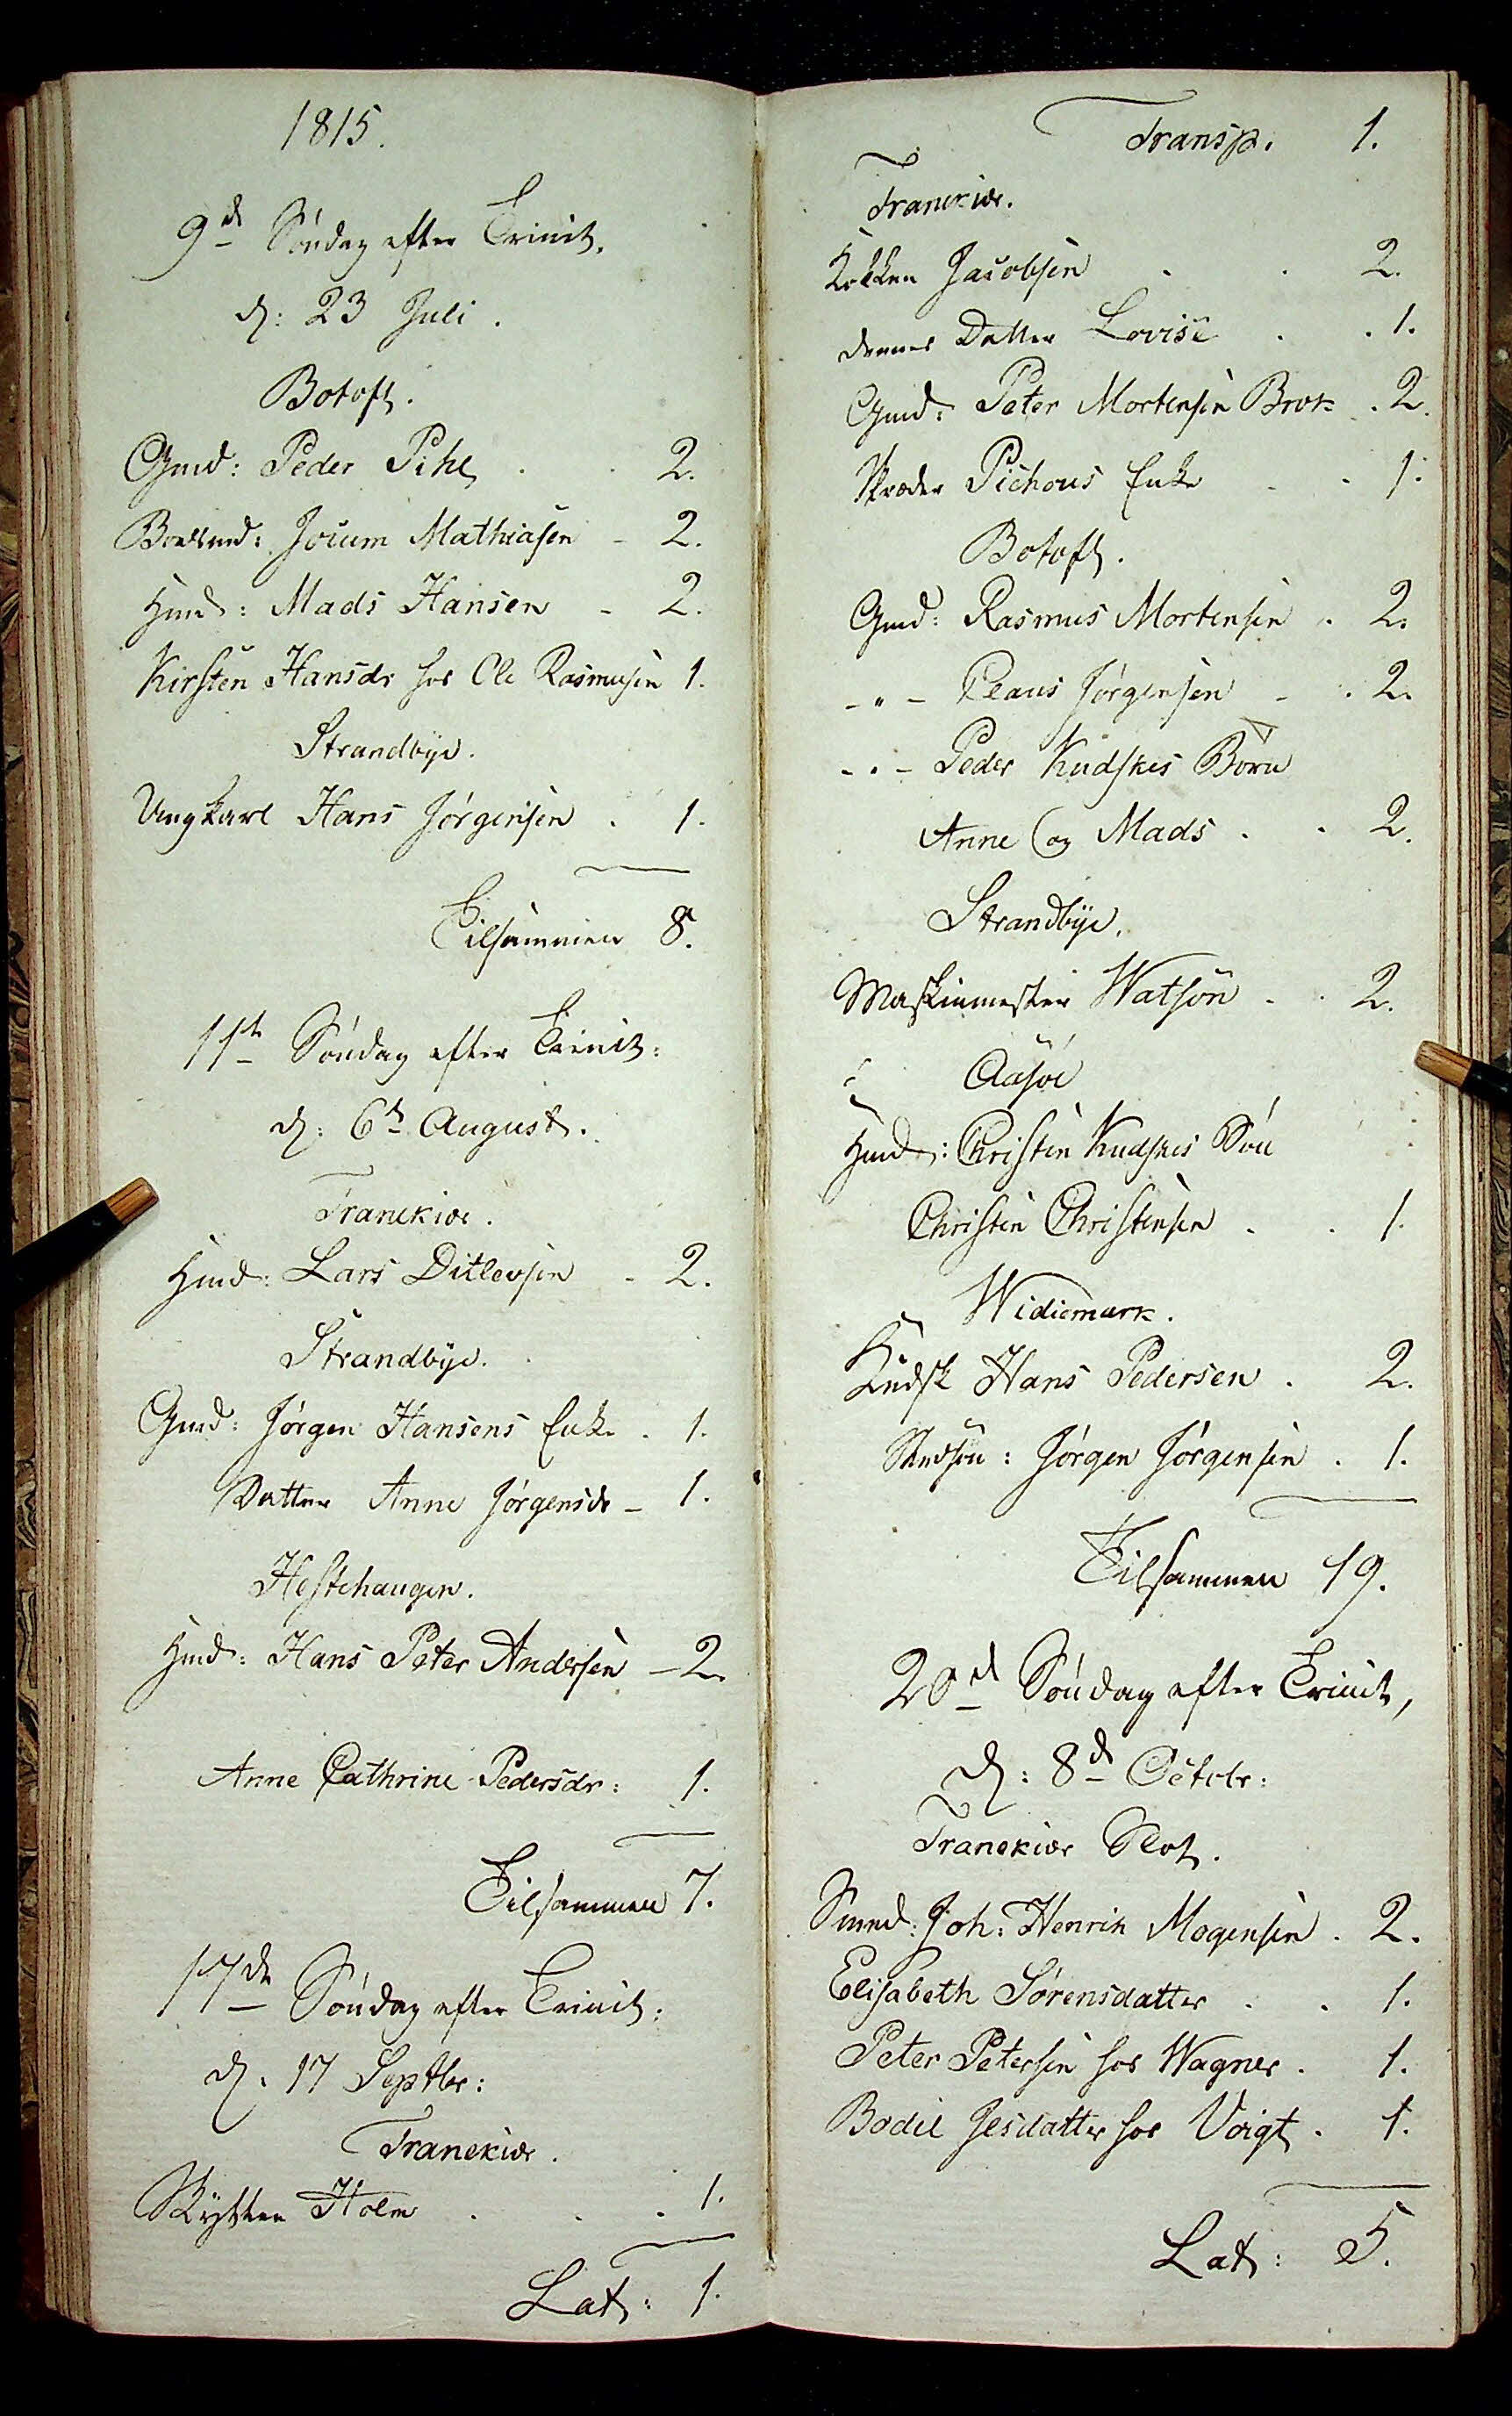1815.
9de Søndag efter Trinit.
d: 23 Juli.
Botoft
Gmd: Peder Pihl . . 2.
Boelsmd: Jocum Mathiasen - 2.
Hmd: Mads Hansen - 2.
Kirsten Hansdr hos Ole Rasmusen 1.
Strandbye.
Ungkarl Hans Jørgensen .1
Tilsammen 8.
11te Søndag efter Trinit:
d: 6te August.
Tranekiær
Hmd: Lars Ditlevsen - 2.
Strandbye
Gmd: Jørgen Hansens Enke . 1.
Datter Anne Jørgensdr -1.
Hestehaugen.
Hmd: Hans Pater Andersen - 2
Anne Cathrine Pedersdr: 1
Tilsammen 7.
17de Søndag efter Trinit:
d: 17 Septbr:
Tranekiær.
Skytten Holm . . . 1.
Lat: 1
Transp: 1.
Tranekiær.
Kokken Jacobsen . . 2.
deres Datter Lovises . . 1.
Gmd: Peder Mortensen Brok . 2
Skræder Pichous Enke . . 1.
Botoft
Gmd: Rasmus Mortensen . 2.
-"- Claus Jørgensen - . 2.
-"- Peder Kudskes Børn
Anne og Mads . . 2.
Strandbye.
Maskinmester Watson . . 2.
Aasøe
Hmd: Christen Kudskes Søn
Christen Christensen . . 1.
Widiemark.
Kudsk Hans Pedersen . 2.
Stedsøn: Jørgen Jørgensen . 1.
Tilsammen 19.
20de Søndag efter Trinit,
d: 8de Octobr:
Tranekiær Slot.
Smed: Joh: Henrik Mogensen . 2.
Elisabeth Sørensdatter . . 1.
Peter Petersen hos Wagner . 1.
Bodil Jesdatter hos Voigt . 1.
Lat: 5.
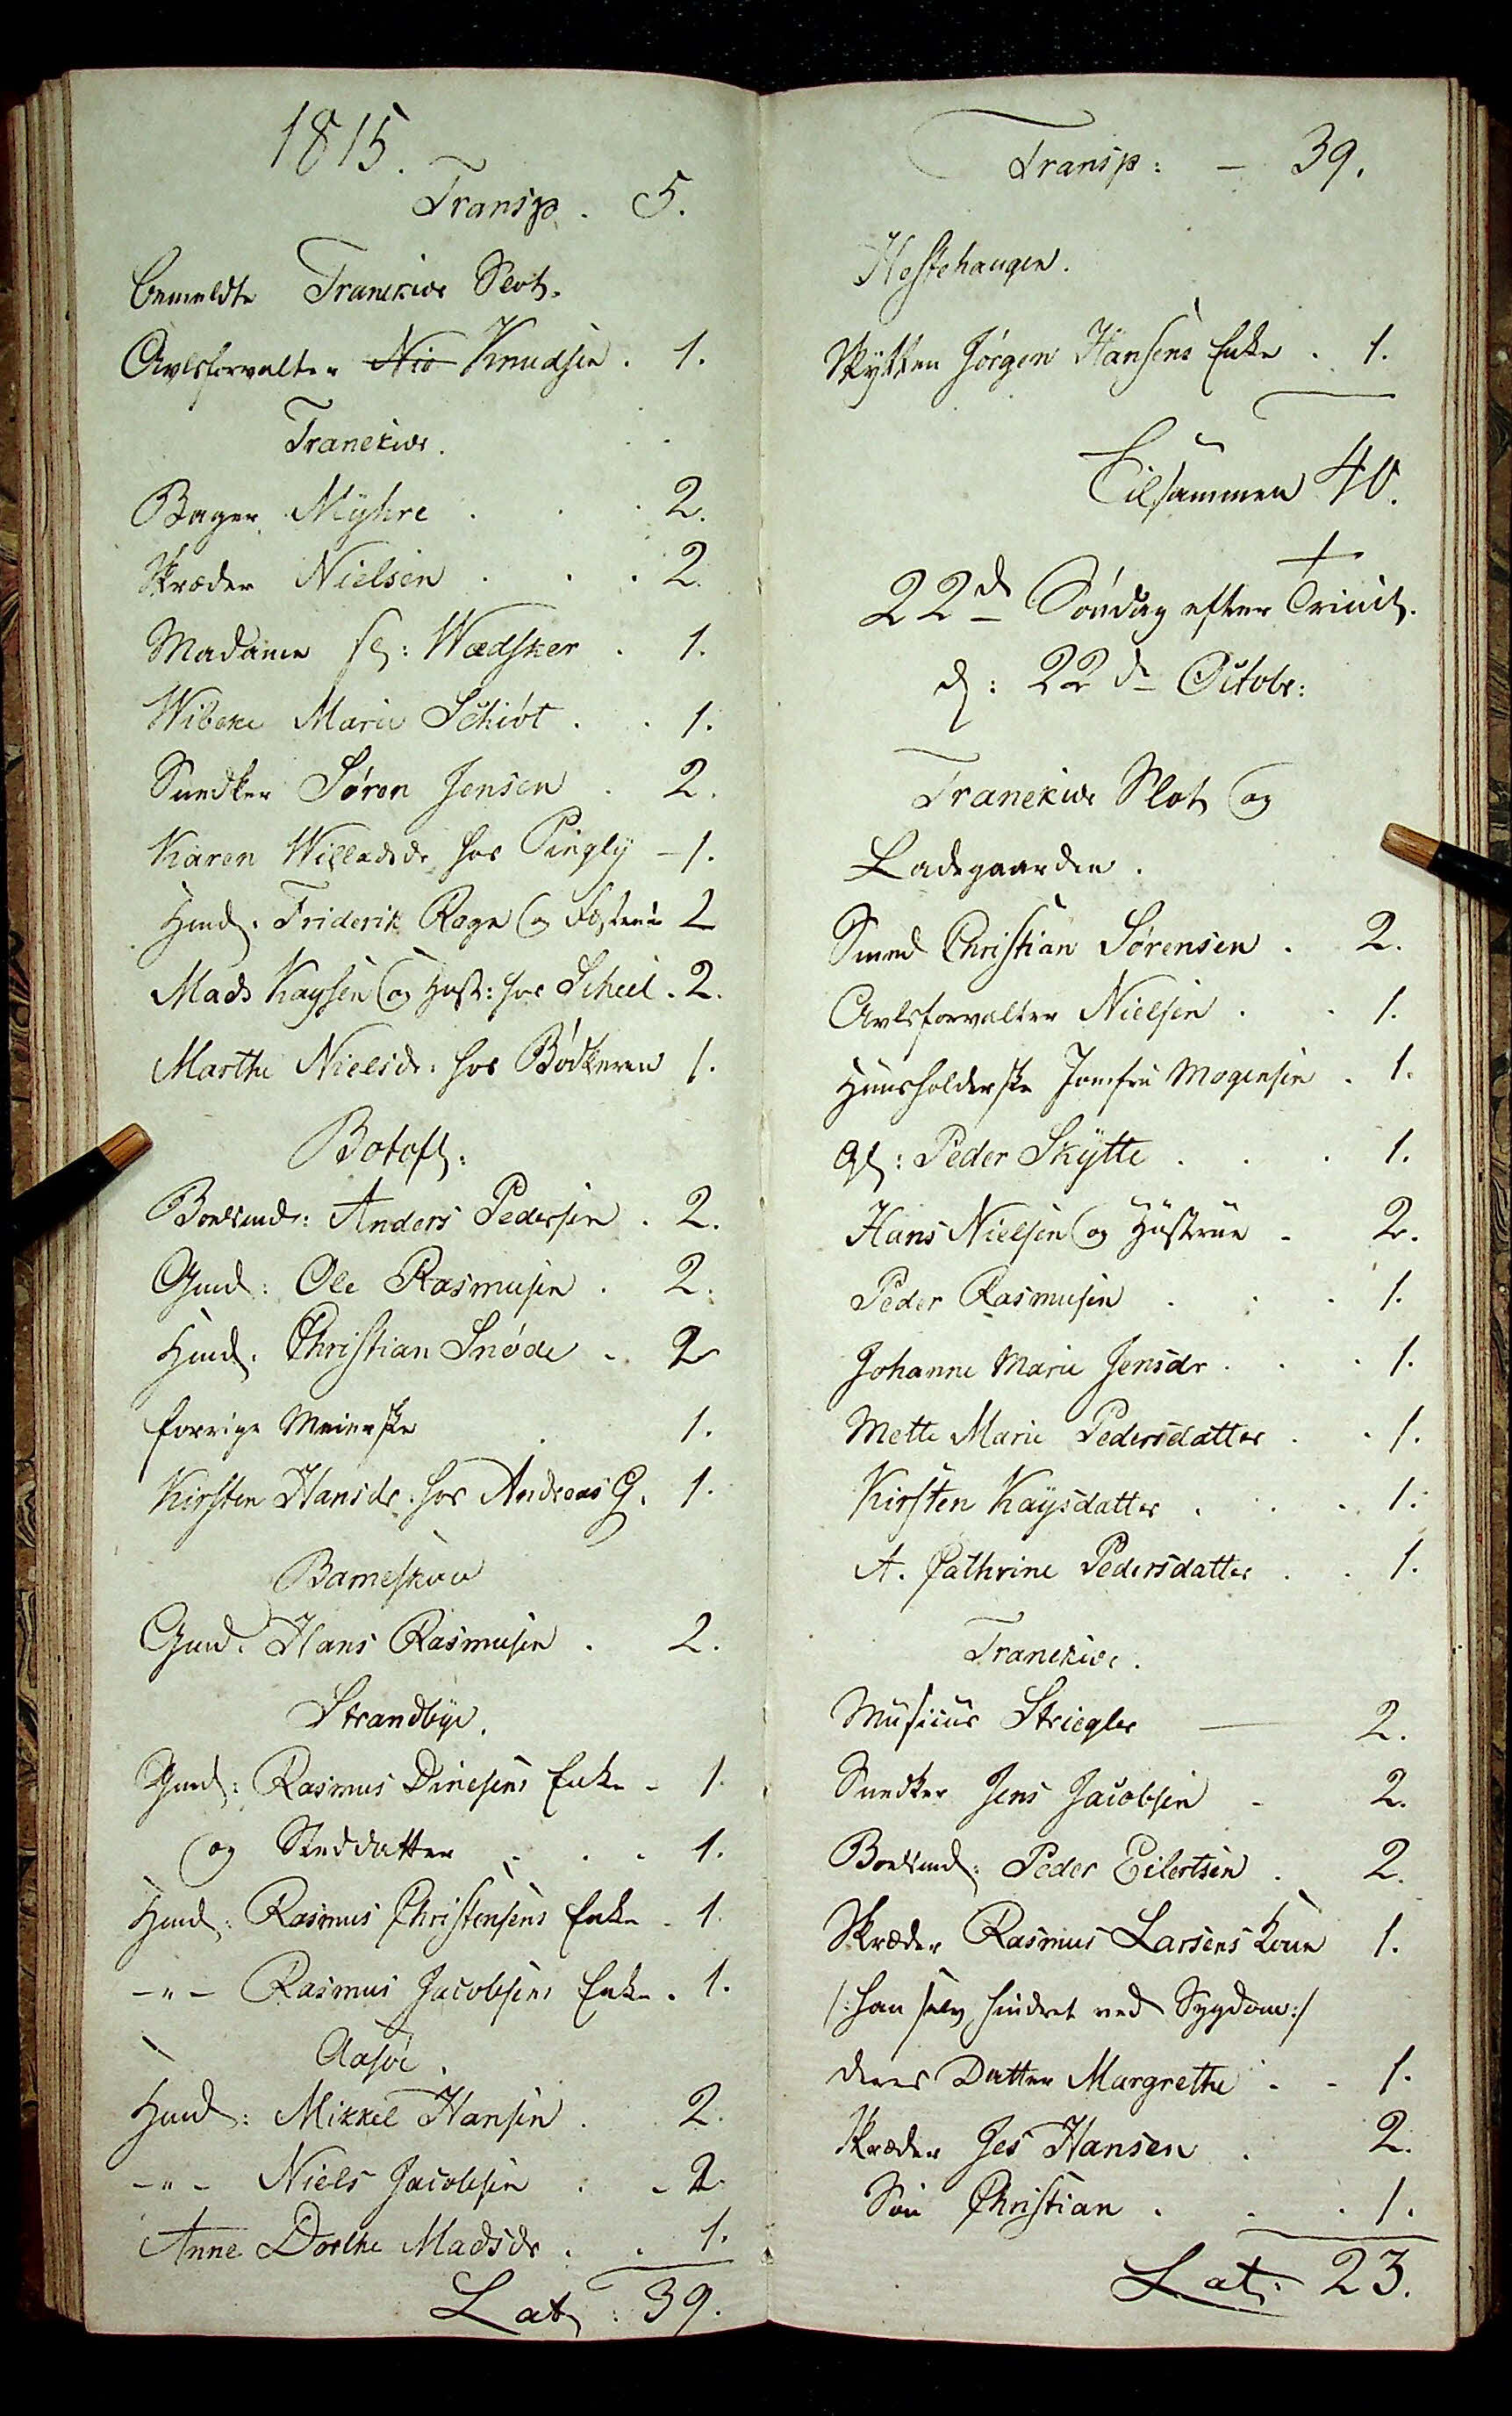1815
Transp . 5.
bemeldte Tranekiær Slot.
Avlsforvalter Nie Knudsen . 1.
Tranekiær.
Bager Myhre . . . 2.
Skræder Nielsen . . . 2.
Madame sl: Wædsker . 1.
Wibeke Marie Schiøt . . 1.
Snedker Søren Jensen . 2.
Karen Willadsdr hos Pingly - 1.
Hmd: Friderik Rogn og Hustrue 2
Mads Kaysen og Hust: hos Scheel . 2.
Marthe Nielsdr: hos Bødkeren 1.
Botoft
Boelsmd: Anders Pedersen . 2.
Gmd: Ole Rasmusen . 2
Hmd. Christian Snøde - . 2
forrige Meierske . 1.
Kirsten Hansdr: hos Anders G . 1.
Bameskov
Gmd. Hans Rasmusen . 2
Strandbye.
Gmd: Rasmus Dinesens Enke - 1.
og Steddatter . . . 1.
Hmd: Rasmus Christensens Enke - 1.
-"- Rasmus Jacobsens Enke . 1.
Aasør
Hmd: Mikkel Hansen . . 2.
-"- Niels Jacobsen 2 
Anne Dorthe Madsdr . . 1.
Lat: 39.
Transp: - 39.
Hestehaugen.
Skytten Jørgen Hansens Enke . 1.
Tilsammen 40.
22de Søndag efter Trinit.
d: 20de Octobr:
Tranekiær Slot og
Ladegaarden
Smed Christian Sørensen . 2.
Avlsforvalter Nielsen . . 1.
Huusholderse Jomfru Mogensen . 1.
gl: Peder Skytte . . . 1.
Hans Nielsen og Hustrue . 2.
Peder Rasmusen . . . 1.
Johanne Marie Jensdr . . . 1.
Mette Marie Pedersdatter . . 1
Kirsten Kaysdatter . . . 1.
A. Cathrine Pedersdatter . . 1.
Tranekiær
Musicus Striegler - 2.
Sneder Jens Jacobsen - 2.
Boelsmd: Peder Eilertsen . 2.
Skræder Rasmus Larsens Kone 1.
/: han selv hindet ved Sygdom :/
deres Datter Margrethe . . 1.
Skræder Jes Hansen . 2.
Søn Christian . . . 1.
Lat: 23.